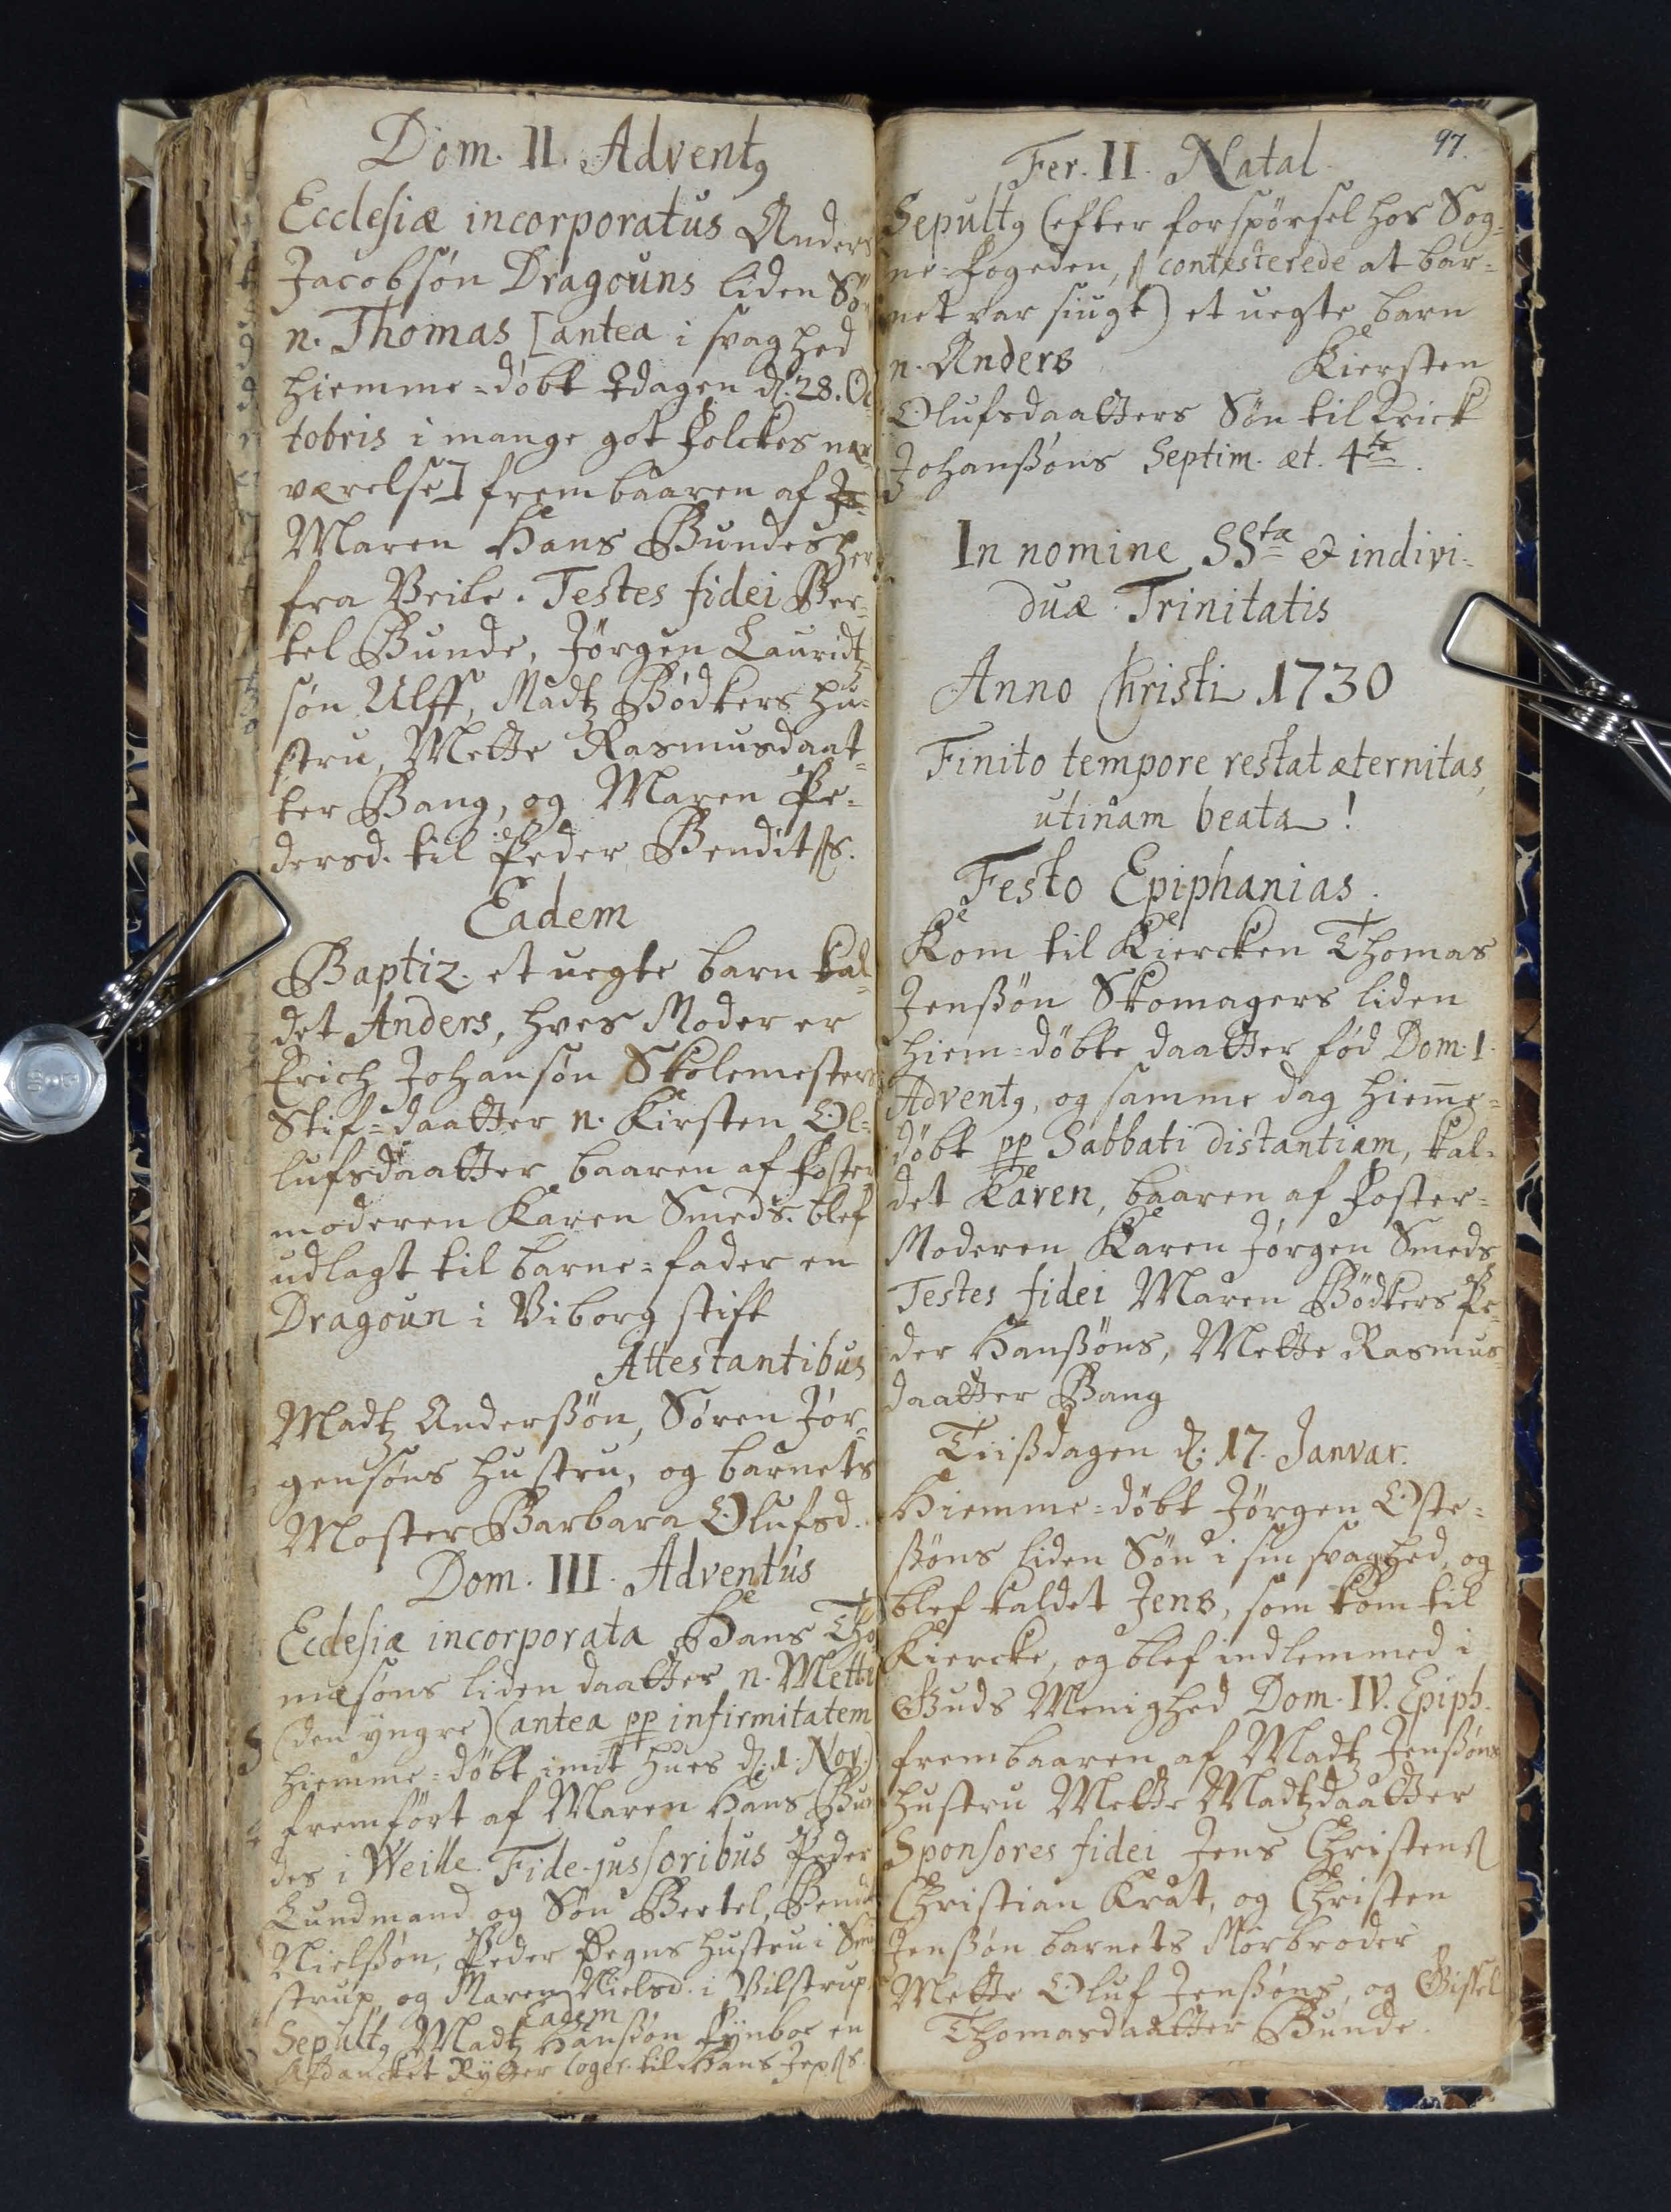Dom. II. Adventq
Ecclesiæ incorporatus Anders
Jacobsøn Dragouns liden Søn
n. Thomas [antea i svaghed
hiemme=døbt d. 28 Oc
tobris i mange got folkes nær
værelse] frembaaren af
Maren Hans Bundes her
fra Veile. Testes fodei Ber¬
tel Bunde, Jørgen Lauridtz¬
søn Ulff, Madtz Bødkers hu¬
stru, Mette Rasmusdaat¬
ter Bang, og Maren Pe¬
dersd. til Peder Benditss.
Eadem
Baptiz. et uegte barn kal¬
det Anders, hves Moder er
Erich Johansøn Skolemesters
Stif=daatter n. Kirsten Ol¬
lufsdaatter baaren af Foster
moderen Karen Smeds. blef
udlagt til barne=fader en
Dragoun i Viborg stift
Attestantibus
Madtz Annderssøn, Søren Jør¬
gensøns hustru, og barnets
Moster Barbara Olufsd.
Dom. III. Adventus
Ecclesiæ incorporata Hans Tho¬
mæsøns liden daatter n. Mette
(den yngre) (antea pp infirmitatem
hiemme=døbt i mit hues d. 1. Nov.)
fremført af Maren Hans Bun
des i Weille. Fide-jusoribus Peder
Lundmand og Søn Bertel,
Nielssøn. Peder Degns hustru i Smids
strup, og Maren Nielsd. i Vilstrup
Eadem
Sepultq Madtz Hanssøn Fynboe en
Afdancket Rytter loger. til Hans Jepss.
97.
Fer. II. Natal
Sepultq (efter forspørsel hos Sog¬
ne=Fogeden, ## contesterede at bar¬
net var siugt) et uegte barn
n. Anders
Kiersten
Olufsdaatters Søn til Erick
Johanssøns Septim. æt. 4##
In nomine SS## et indivi¬
duæ. Trinitatis
Anno Christi 1730
Finito tempore restat æternitas
utinam beata
Festo Epiphanias
Kom til Kiercken Thomas
Jenssøn Skomagers liden
hiem=døbte daatter fød Dom. I
Adventq, og samme dag hiemme¬
døbt pp Sabbati distantiam, kal¬
det Karen, baaren af Foster¬
Moderen Karen Jørgen Smeds
Testes fedei Maren Bødkers Pe¬
der Hanssøns, Mette Rasmus¬
daatter Bang
Tiissdagen d: 17. Janvar.
Hiemme=døbt Jørgen Oste¬
ssøns liden Søn i sin svaghed, og
blef kaldet Jens, som kom til
Kiercke, og blef indlemmed i
Guds Menighed Dom. IV. Epiph.
frembaaren af Madtz Jenssøns
hustru Mette Madtzdaatter
Sponsores fidei Jens Christens
Christian Krat, og Christen 
Jenssøn barnets Morbroder
Mette Oluf Jenssøns, og Gissel
Thomasdaatter Bunde